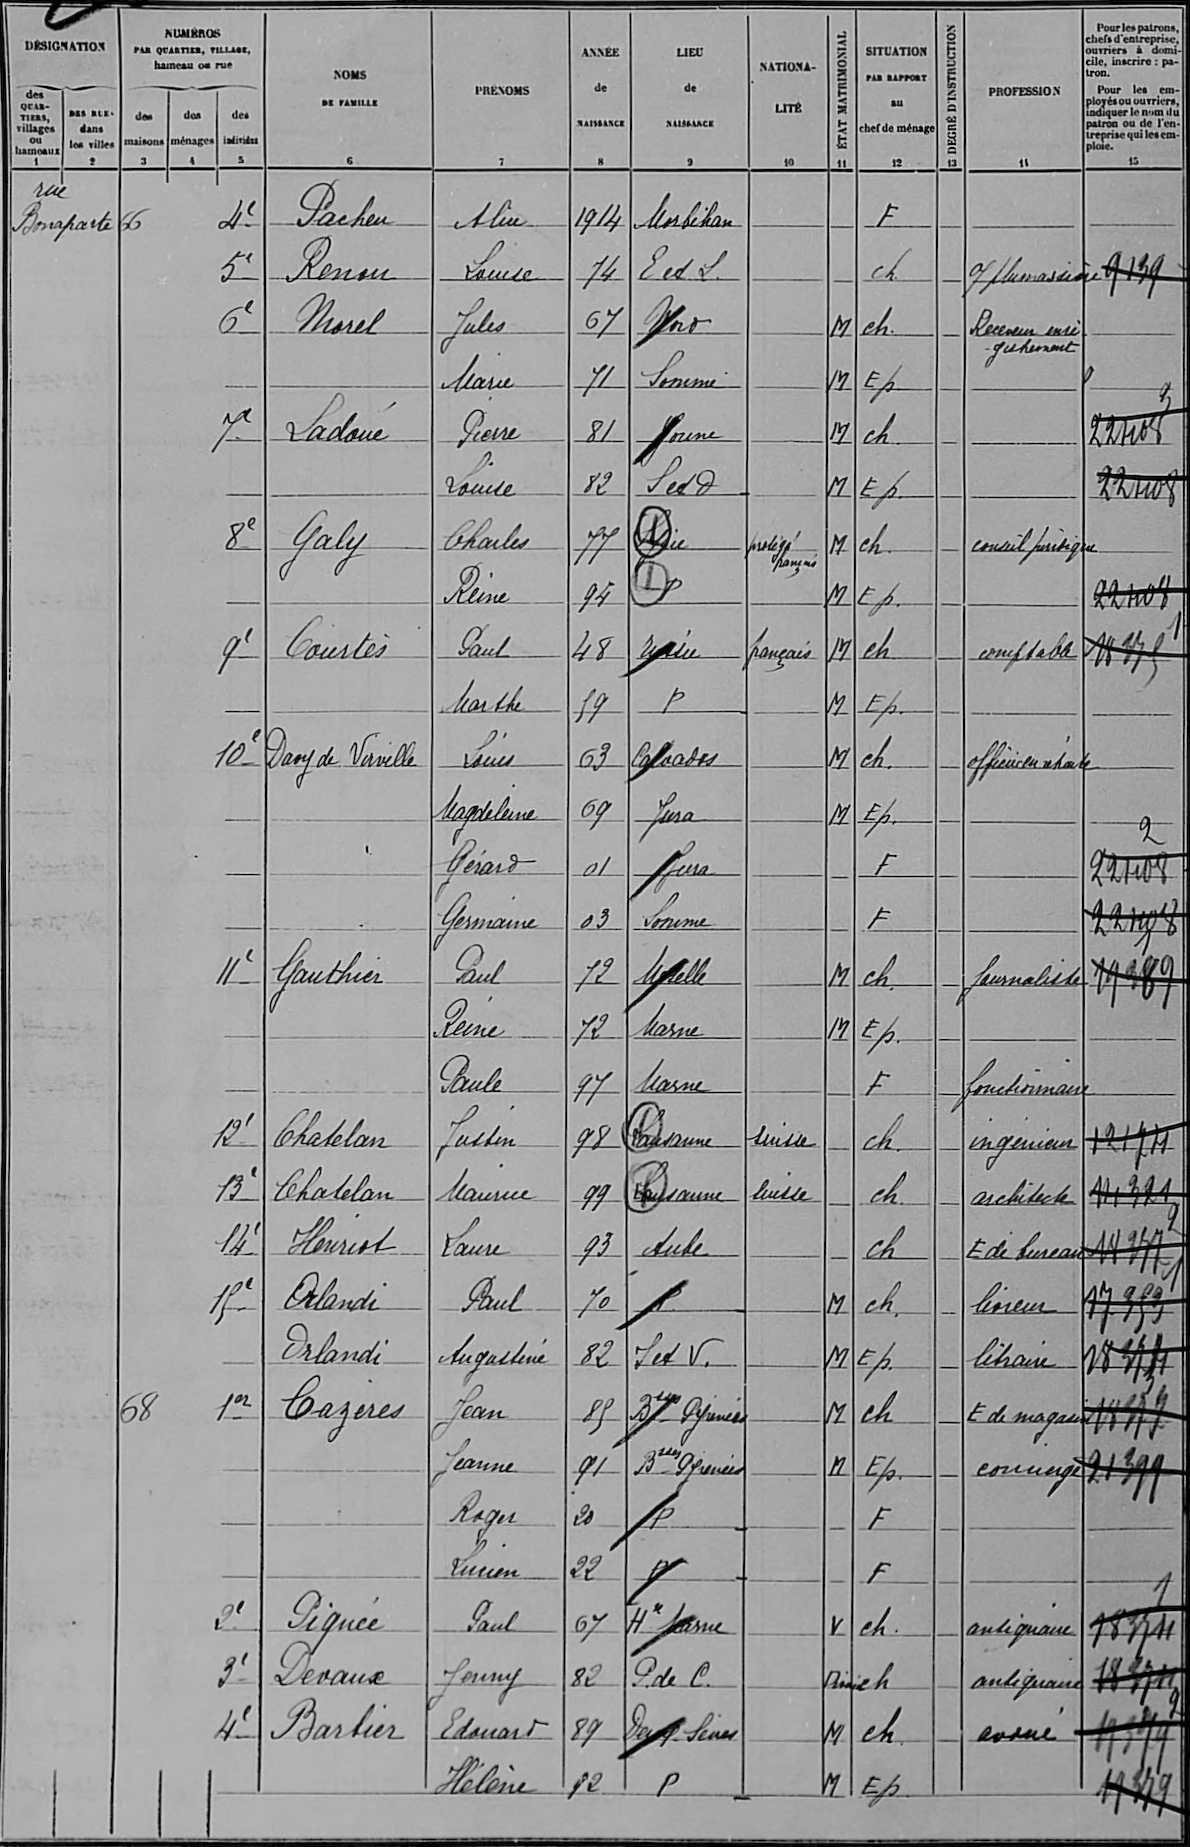Pacheu/Aline/1914/Morbihan/¤/¤/F/¤/¤/¤
Renon/Louise/74/E et L /¤/¤/ch /¤/o plumassière/9139
Morel/Jules/67/nord/¤/M/ch /¤/Receveur enre!gistrement/¤
¤/Marie/71/Somme/¤/M/Ep/¤/¤/¤
Ladoué/Pierre/81/Yonne/¤/M/ch /¤/¤/22408
¤/Louise/82/S et O/¤/M/Ep/¤/¤/22408
Galy/Charles/77/Syrie/protégé français/M/ch /¤/conseil juridique/¤
¤/Rein/94/P/¤/M/Ep /¤/¤/22408
Courtès/Paul/48/Russie/français/M/ch/¤/comptable/18375
¤/Marthe/59/P/¤/M/Ep/¤/¤/¤
Daoy de Virville/Louis/63/Calvados/¤/M/ch /¤/officier retraite/¤
¤/MAgdeleine/69/Jura/¤/M/Ep /¤/¤/¤
¤/Gérard/01/Jura/¤/¤/F/¤/¤/22408
¤/Germaine/03/Somme/¤/¤/F/¤/¤/22408
Gauthier/Paul/72/Moselle/¤/M/ch/¤/journaliste/19389
¤/Reine/72/Marne/¤/M/Ep /¤/¤/¤
¤/Paule/97/Marne/¤/¤/F/¤/fonctionnaire/¤
Chatelan/Justin/98/Lausanne/Suisse/¤/ch /¤/ingénieur/12174
Chatelan/Maurice/99/Lausanne/Suisse/¤/ch /¤/architecte/14321
Henriot/Laure/93/Aube/¤/¤/ch/¤/E de bureau/18377
Orlandi/Paul/70/P/¤/M/ch/¤/livreur/17353
Orlandi/Augustine/82/I et V /¤/M/Ep /¤/libraire/18374
Cazères/Jean/85/Bsses Pyrénées/¤/M/ch/¤/E de magasin/18377
¤/Jeanne/91/Bsses Pyrénées/¤/M/Ep /¤/concierge/21399
¤/Roger/20/P/¤/¤/F/¤/¤/¤
¤/Lucien/22/P/¤/¤/F/¤/¤/¤
Pignée/paul/67/Hte Marne/¤/V/ch /¤/antiquaire/18374
Devaux/Jenny/82/P de C /¤/Div/ch/¤/antiquaire/18374
Barbier/Edouard/89/Deux-Sèvres/¤/M/ch/¤/avoué/19379
¤/Hélène/92/P/¤/M/Ep/¤/¤/19379
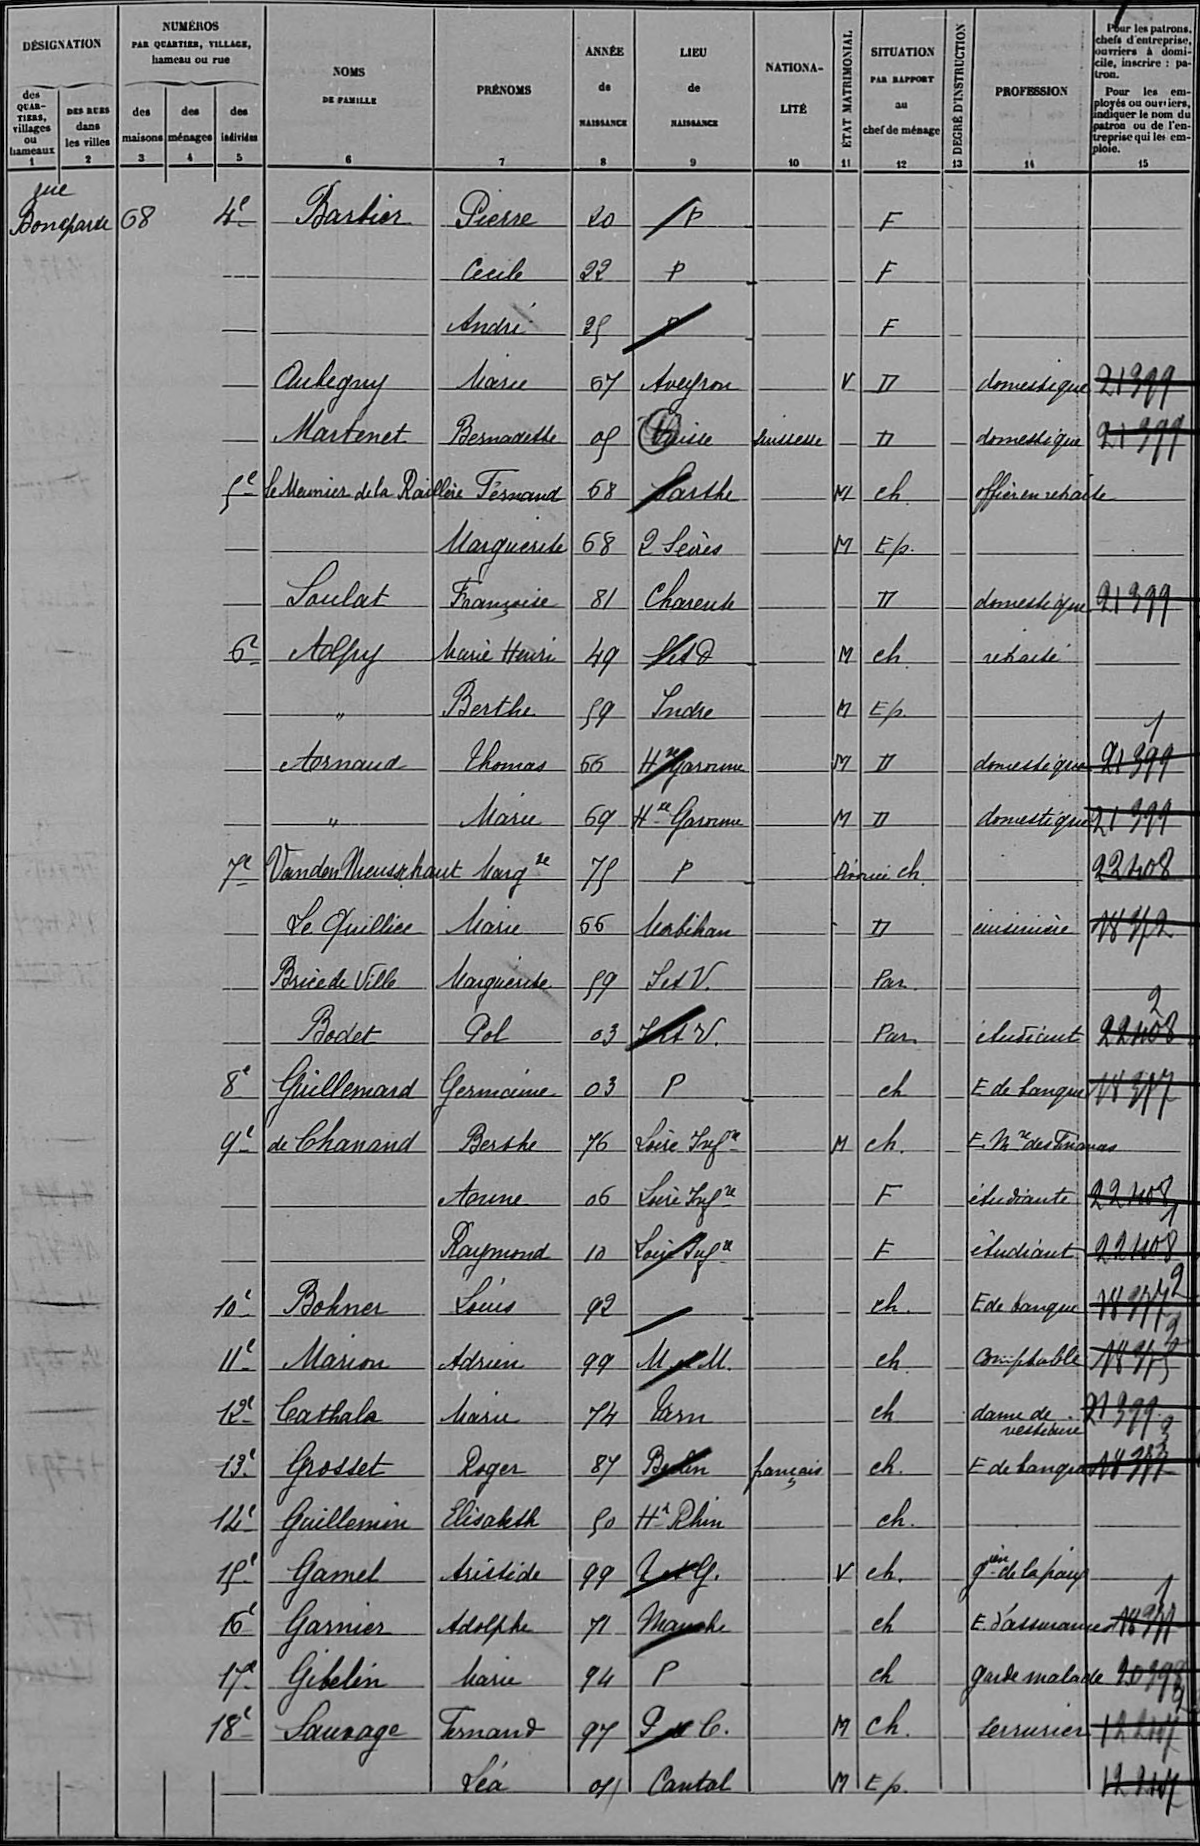Barbier/Pierre/20/P/¤/¤/F/¤/¤/¤
¤/Cécile/22/P/¤/¤/F/¤/¤/¤
¤/André/25/P/¤/¤/F/¤/¤/¤
Aubegrey/MArie/07/Aveyron/¤/V/D/¤/domestique/21399
MArtenet/Bernadette/05/Suisse/suissesse/¤/D/¤/domestique/21399
Le Meunier de la Raillère/Fernand/68/Sarthe/¤/M/ch/¤/officier en retraite/¤
¤/Marguerite/68/2 sèvres/¤/M/Ep /¤/¤/¤
Saulat/Françoise/81/Charente/¤/¤/D/¤/domestique/21399
Alpy/Marie Henry/49/S et O/¤/M/ch/¤/retraité/¤
"/Berthe/59/Indre/¤/M/Ep /¤/¤/¤
Arnaud/Thomas/66/Hte Garonne/¤/M/D/¤/domestique/21399
"/Marie/69/Hte Garonne/¤/M/D/¤/domestique/21399
Vanden Neusrhaut/Margre/75/P/¤/Divorcée/ch/¤/¤/22408
Le Quillice/Marie/66/Morbihan/¤/¤/D/¤/cuisinière/18372
Brice de Ville/Marguertie/89/I et V /¤/¤/Par /¤/¤/¤
Bodet/pol/03/I et V /¤/¤/Par/¤/étudiant/22408
Guillemard/germaine/03/P/¤/¤/ch/¤/E de banque/18317
de Chanand/berthe/76/loire Infre/¤/M/ch /¤/E Mre des Finances/¤
¤/Arine/06/loire Infre/¤/¤/F/¤/étudiante/22408
¤/Raymond/10/Loire Infre/¤/¤/F/¤/étudiant/22408
Bohner/Louis/92/¤/¤/¤/ch /¤/E de banque/18377
Marion/Adiren/99/M et M /¤/¤/ch/¤/comptable/18375
Cathala/Marie/74/Tarn/¤/¤/ch/¤/dame de necessaire/21399
Grosset/Roger/87/Berlin/français/¤/ch/¤/E de banque/18377
Guillemin/Elisabeth/50/Ht Rhin/¤/¤/ch/¤/¤/¤
Gamùel/aristide/99/L et G /¤/V/ch /¤/gien de la paix/¤
Garnier/Adolphe/71/manche/¤/¤/ch/¤/E d'assurances/18371
Gibelin/Marie/94/P/¤/¤/ch/¤/garde malade/20398
Sauvage/Fernand/97/P de C /¤/M/ch /¤/serrurier/12247
¤/Léa/05/Cantal/¤/M/Ep/¤/¤/12247